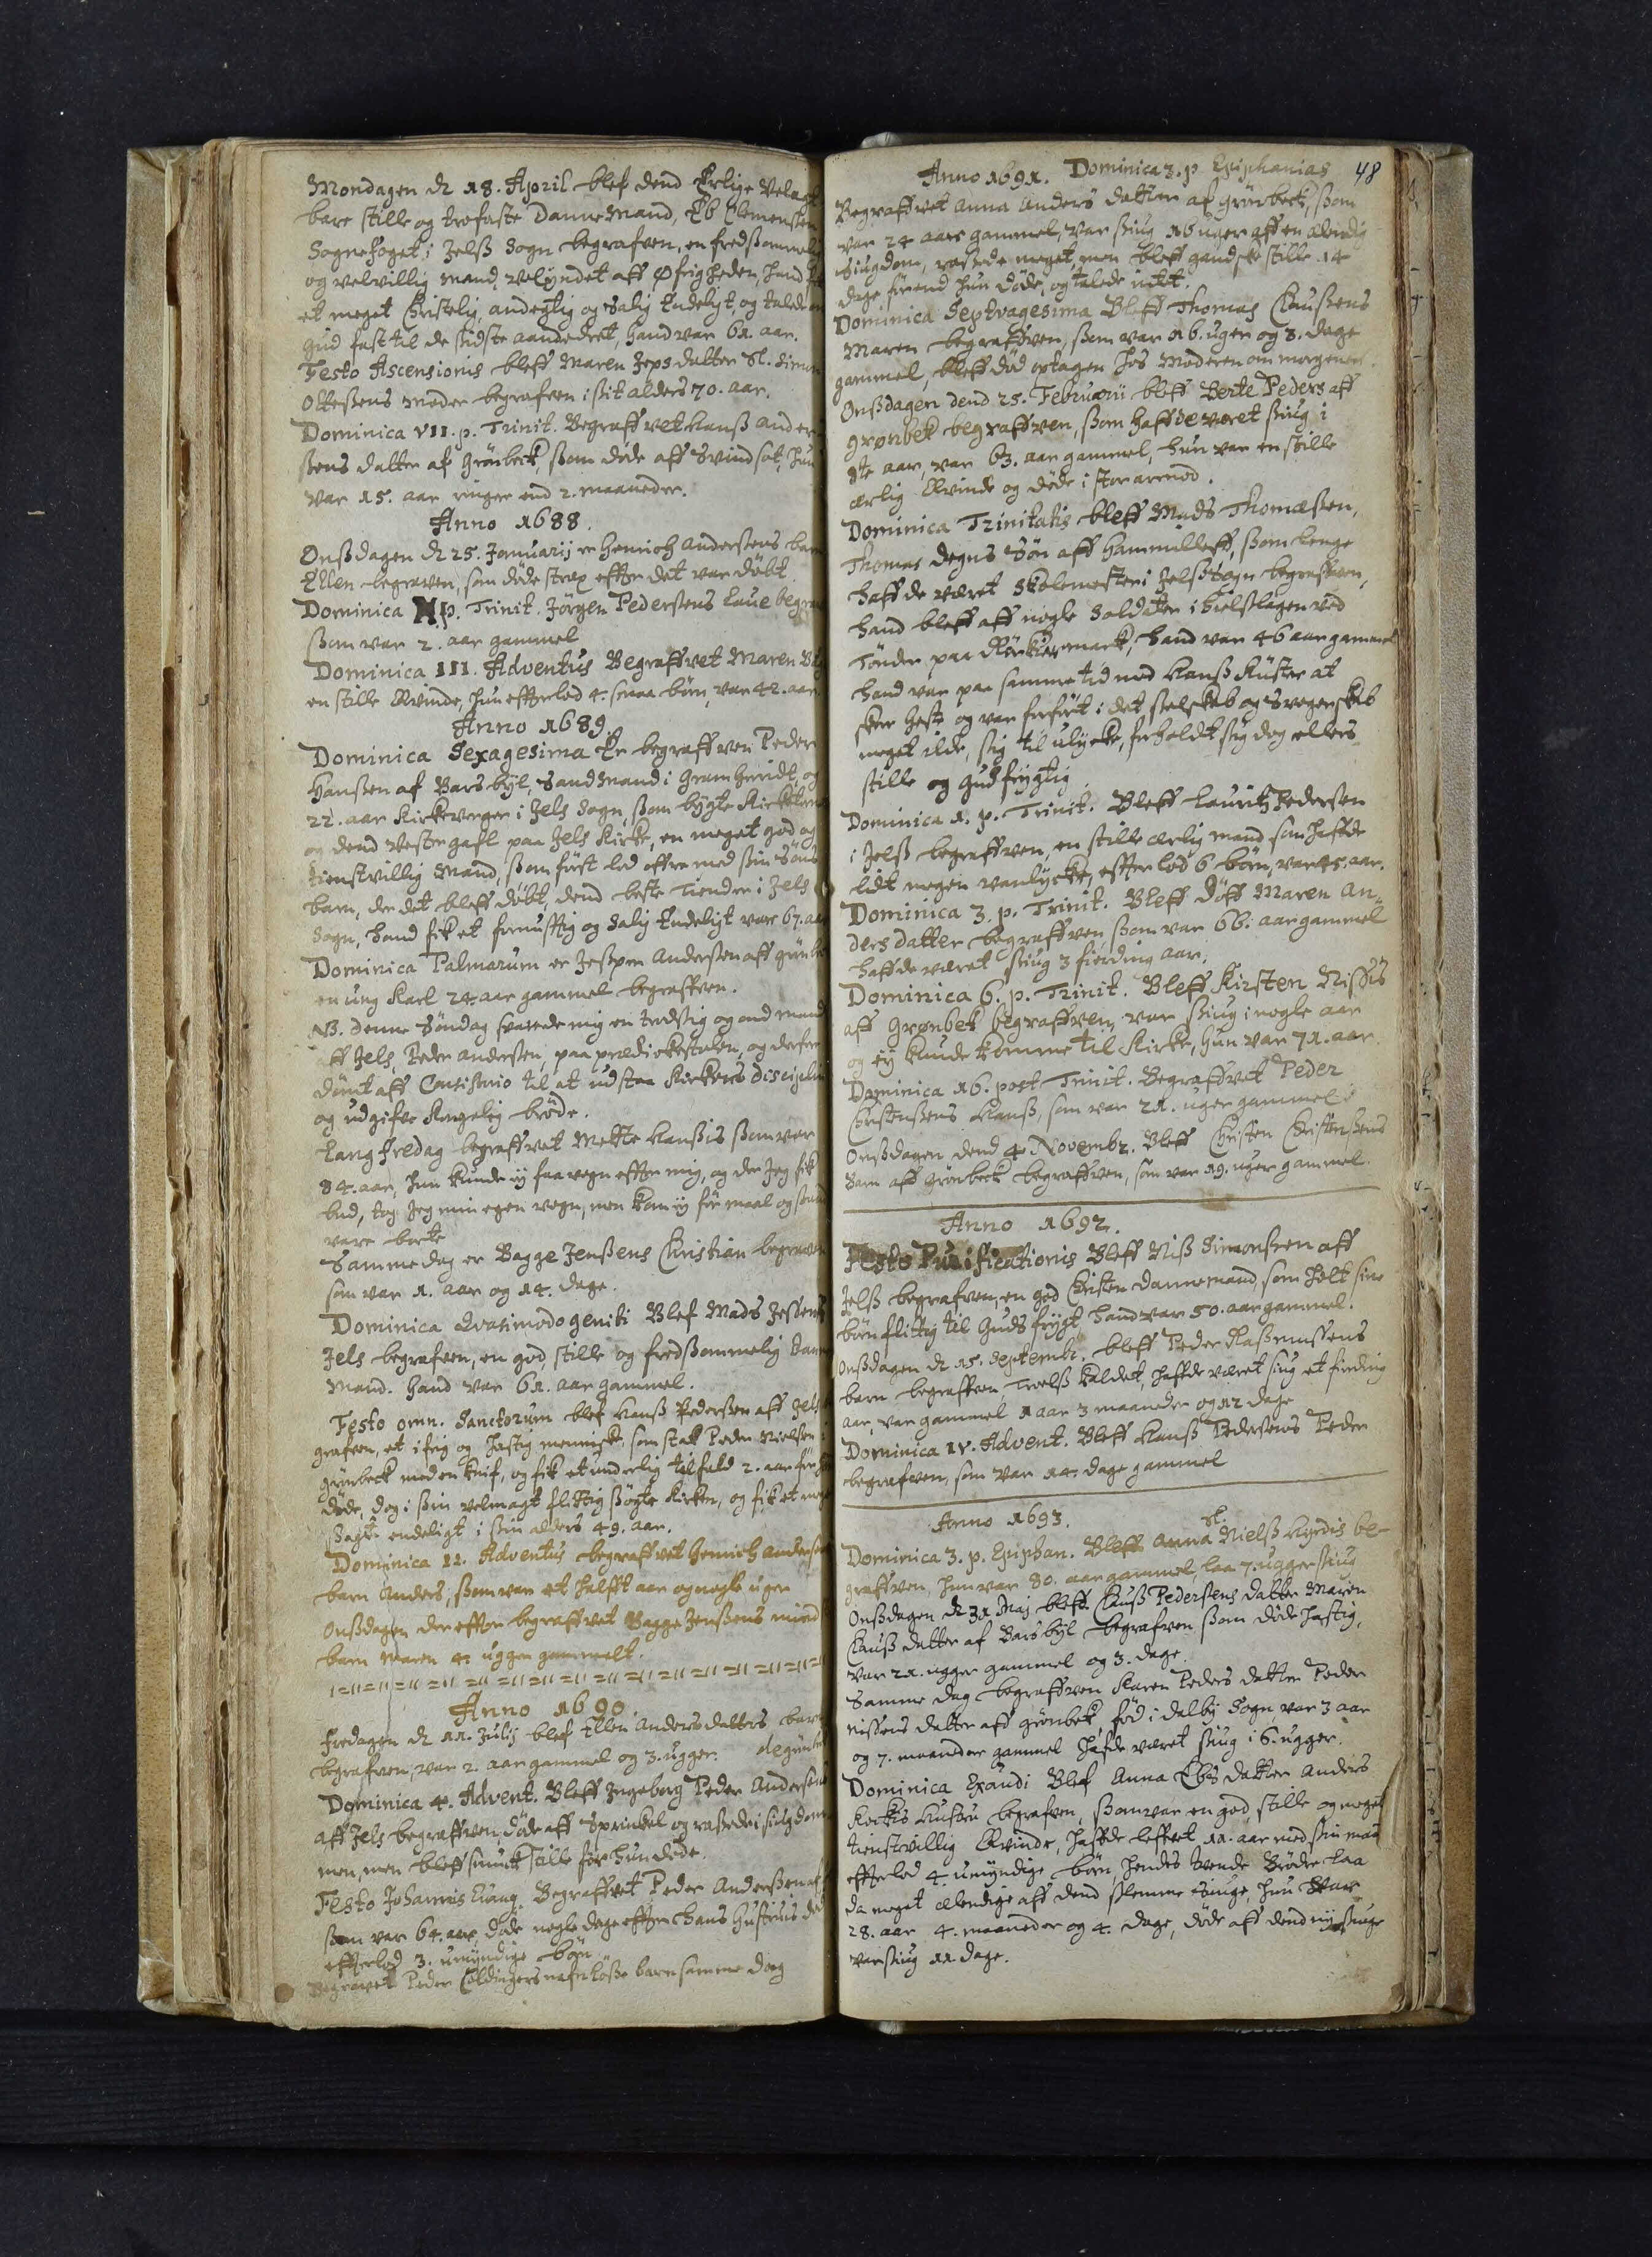Mondagen d 18. April blef dend Erlige Velagt
bare stille og trofaste Dannemand, Eb Clemenssen
Sognefoget i Jelss Sogn begrafven, en fredssommelig
og velvillig mand, velyndet aff Øfrigheden, hand fik
et meget Christelig, andegtig og salig Endeligt, og kalede
gud fast til de sidste aandedret, Hand var 62. aar.
Festo Ascensions bleff Maren Jeps datter Sl. Simon
Ottessens moder begrafven i ssit alders 70. aar.
Dominica VII. P. Trinit. Begraffvet Hanss Ander
ssens datter af Grønbeck, som døde aff Svindsot, hun
var 15. aar ringere end 2 maaneder.
Anno 1688.
Onssdagen d 25 Januarij er Henrich Andersens barn
Ellen begraven, som døde strax efter det var døbt.
Dominica X p. Trinit Jørgen Pedersens Laue begrav
ssom var 2. aar gammel
Dominica III. Adventis Begraffvet Maren Bag 
en stille Qvinde, hun effterlod 4. smaa børn, var 42 aar.
Anno 1689.
Dominica Sexagesima Er begraffven Peder
Hanssen af Barsbyl, Sandmand i Gram Heridt og
22. aar kirkeverger i Jels Sogn, ssom bygte Kirketorn
og dend vestre gafl paa Jels Kirke, en meget god og
tienstvillig Mand, ssom først lod offre med ssin Søns
barn, der det bleff døbt, dend beste tiender i Jels
Sogn, hand fik et fornufftig og salig Endeligt var 67 aa
Dominica Palmarum er Jessper Andersen aff grønbe 
en ung karl 24. aar gammel begraffven.
NB. denne Søndag ##rede mig en trodsig og ond mand
aff Jels, Peder Andersen, paa Prædickestolen, og derfor
dømt aff Consistorio til at udstaa Kirkens disciplin
og udgifter kongelig brøde.
Langfredag begraffvet Mette Hanssis som var
84. aar, hun kunde ey faa vogn efter mig, og da Jeg fik
bud, tog Jeg min egen vogn, men kom ey før maal og
vare borte
Samme dag er Bagge Jenssens Christian begrafven
som var 1. aar og 14. dage.
Dominica Qvasimodogeniti Blef Mads Jessens
Jels begrafven, en god, stille og fredssommelig Danne
mand. Hand var 61. aar gammel.
Festo omn. Sanctorum blef Hanss Pederssen aff Jels
grafven, et ifrig og hastig menniske, som stak Peder Nielsen i
Grønbeck med en knif, og fik et underlig tilfald 2. aar før ha 
døde, dog i ssin velmagt flittig ssøgte kirken, og fik et
sagte endeligt i ssin alders 49. aar.
Dominica II. Adventus begrafvet Henrich Andersens
barn Anders, ssom var et halfft aar og nogle uger
Onsdagen dereffter begraffvet Bagge Jenssens mind
barn Maren 4 ugger gammelt.
Anno 1690
Fredagen d 11. Julij blef Ellen Andersdatters barn
begrafven, var 2. aar gammel og 3. ugger. de Grønbech
Dominica 4. Advent. Bleff Ingeborg Peder Andersens
aff Jels begraffven, døde aff Sprinkel og rassede i siugdom
men, men bleff smuck## Talle før hun døde.
Festo Johannis Euang. Begraffvet Peder Anderssen af
som var 64. aar døde nogle dage effter hans Hustrues død
efterlod 3. umyndige børn
Begravet Peder Coldingers nafnløsse barn samme dag
48
Anno 1691 Dominica 3. p. Epiphanias
Begraffvet Anna Anders datter af Grønbeck, som
var 24 aar gammel, var ssiug 16 uger aff en ælendig
Siugdom, rassede meget, men bleff gandske stille 14
dage førend hun døde, og talede intet.
Dominica Septuagesima Bleff Thomas Clausens
Maren begraffven, som var 16. uger og 3. dage
gammel, bleff død optagen hos moderen om morgenen.
Onssdagen dend 25. Februarii bleff Berte Peders aff
Grønbeck begraffven, ssom haffde været siug i
3te aar, var 63. aar gammel, hun var en stille
ærlig Qvinde og døde i stor armod.
Dominica Trinitatis bleff Mads Thomæssen,
Thomas Degns Søn aff Hammelleff, som lenge
haffde været skolemester i Jelss Sogn begraffven,
hand bleff aff nogle soldater ihielslagen ved
Tønder paa Rørkiermark, hand var 46 aar gammel
Hand var paa samme tid med Hanss Kuster at
Gest og var forført i det selskab og Svogerskab
noget ilde, sig til ulycke, forholdt sig dog ellers
stille og gudfrygtig.
Dominica 1. p. Trinit. Bleff Lauritz Pedersen
i Jelss begraffven, en stille ærlig mand som haffde
lidt megen vanlycke, efterlod 6 børn, var 45. aar.
Dominica 3. p. Trinit. Bleff Døff Maren An
dersdatter begraffven, ssom var 66. aar gammel
hafde veret siug 3 fierdings aar.
Dominica 6. p. Trinit. Bleff Kirsten Nisses
aff Grønbek begraffven, var ssiug i nogle aar
og ey kunde komme til Kirke, hun var 71. aar
Dominica 16. post Trinit. Begraffvet Peder
Christenssens Hanss, som var 21. uger gammel
Onsdagen dend 4. Novembr. Bleff Christen Christensens
barn aff Grønbeck begraffven, som var 19. uger gammel.
Anno 1692
Festo Purifications Bleff Niss Simonssen aff
Jelss begrafven, en god Christen Dannemand, som holt sine
børn flittig til Guds frygt hand var 50. aar gammel.
Onsdagen d. 15. septembr. bleff Peder Rassmusssens
barn begraffven, Troelss kaldet, hafde været siug et fierding
Aar, var gammel 1 aar 3 maaneder og 12 dage
Dominica IV. Advent. Bleff Hanss Pedersens Peder
begrafven, som var 14. Dage gammel
Anno 1693.
Sl.
Dominica 3 p. Epiphan. Bleff Anna Nielss Hyrdis be¬
graffven hun var 80. aar gammel, laa 7. ugger siug
Onsdagen d 31 Maj bleff Clauss Pedersens datter Maren
Clauss Datter af Barsbyl begrafven ssom døde hastig,
var 21. ugger gammel og 3. dage
Samme dag begraffven Karen Peders Datter Peder
Nissens datter aff Grønbek, fød i Dalby Sogn, var 3 aar
og 7. maaneder gammel hafde været ssiug i 6. ugger.
Dominica Exaudi Bleff Anna Ebs datter Anders
Kockis Hustru begrafen, ssom var en god, stille og meget
tienstvillig Qvinde, hafde leffvet 11. aar med sin
efterlod 4. umyndige børn, hendes tvende Brødre laa
da meget ælendige aff dend slemme Siuge, hun var
28. aar 4. maaneder og 4. dage, døde aff dend ny siuge
var siug 11 Dage.